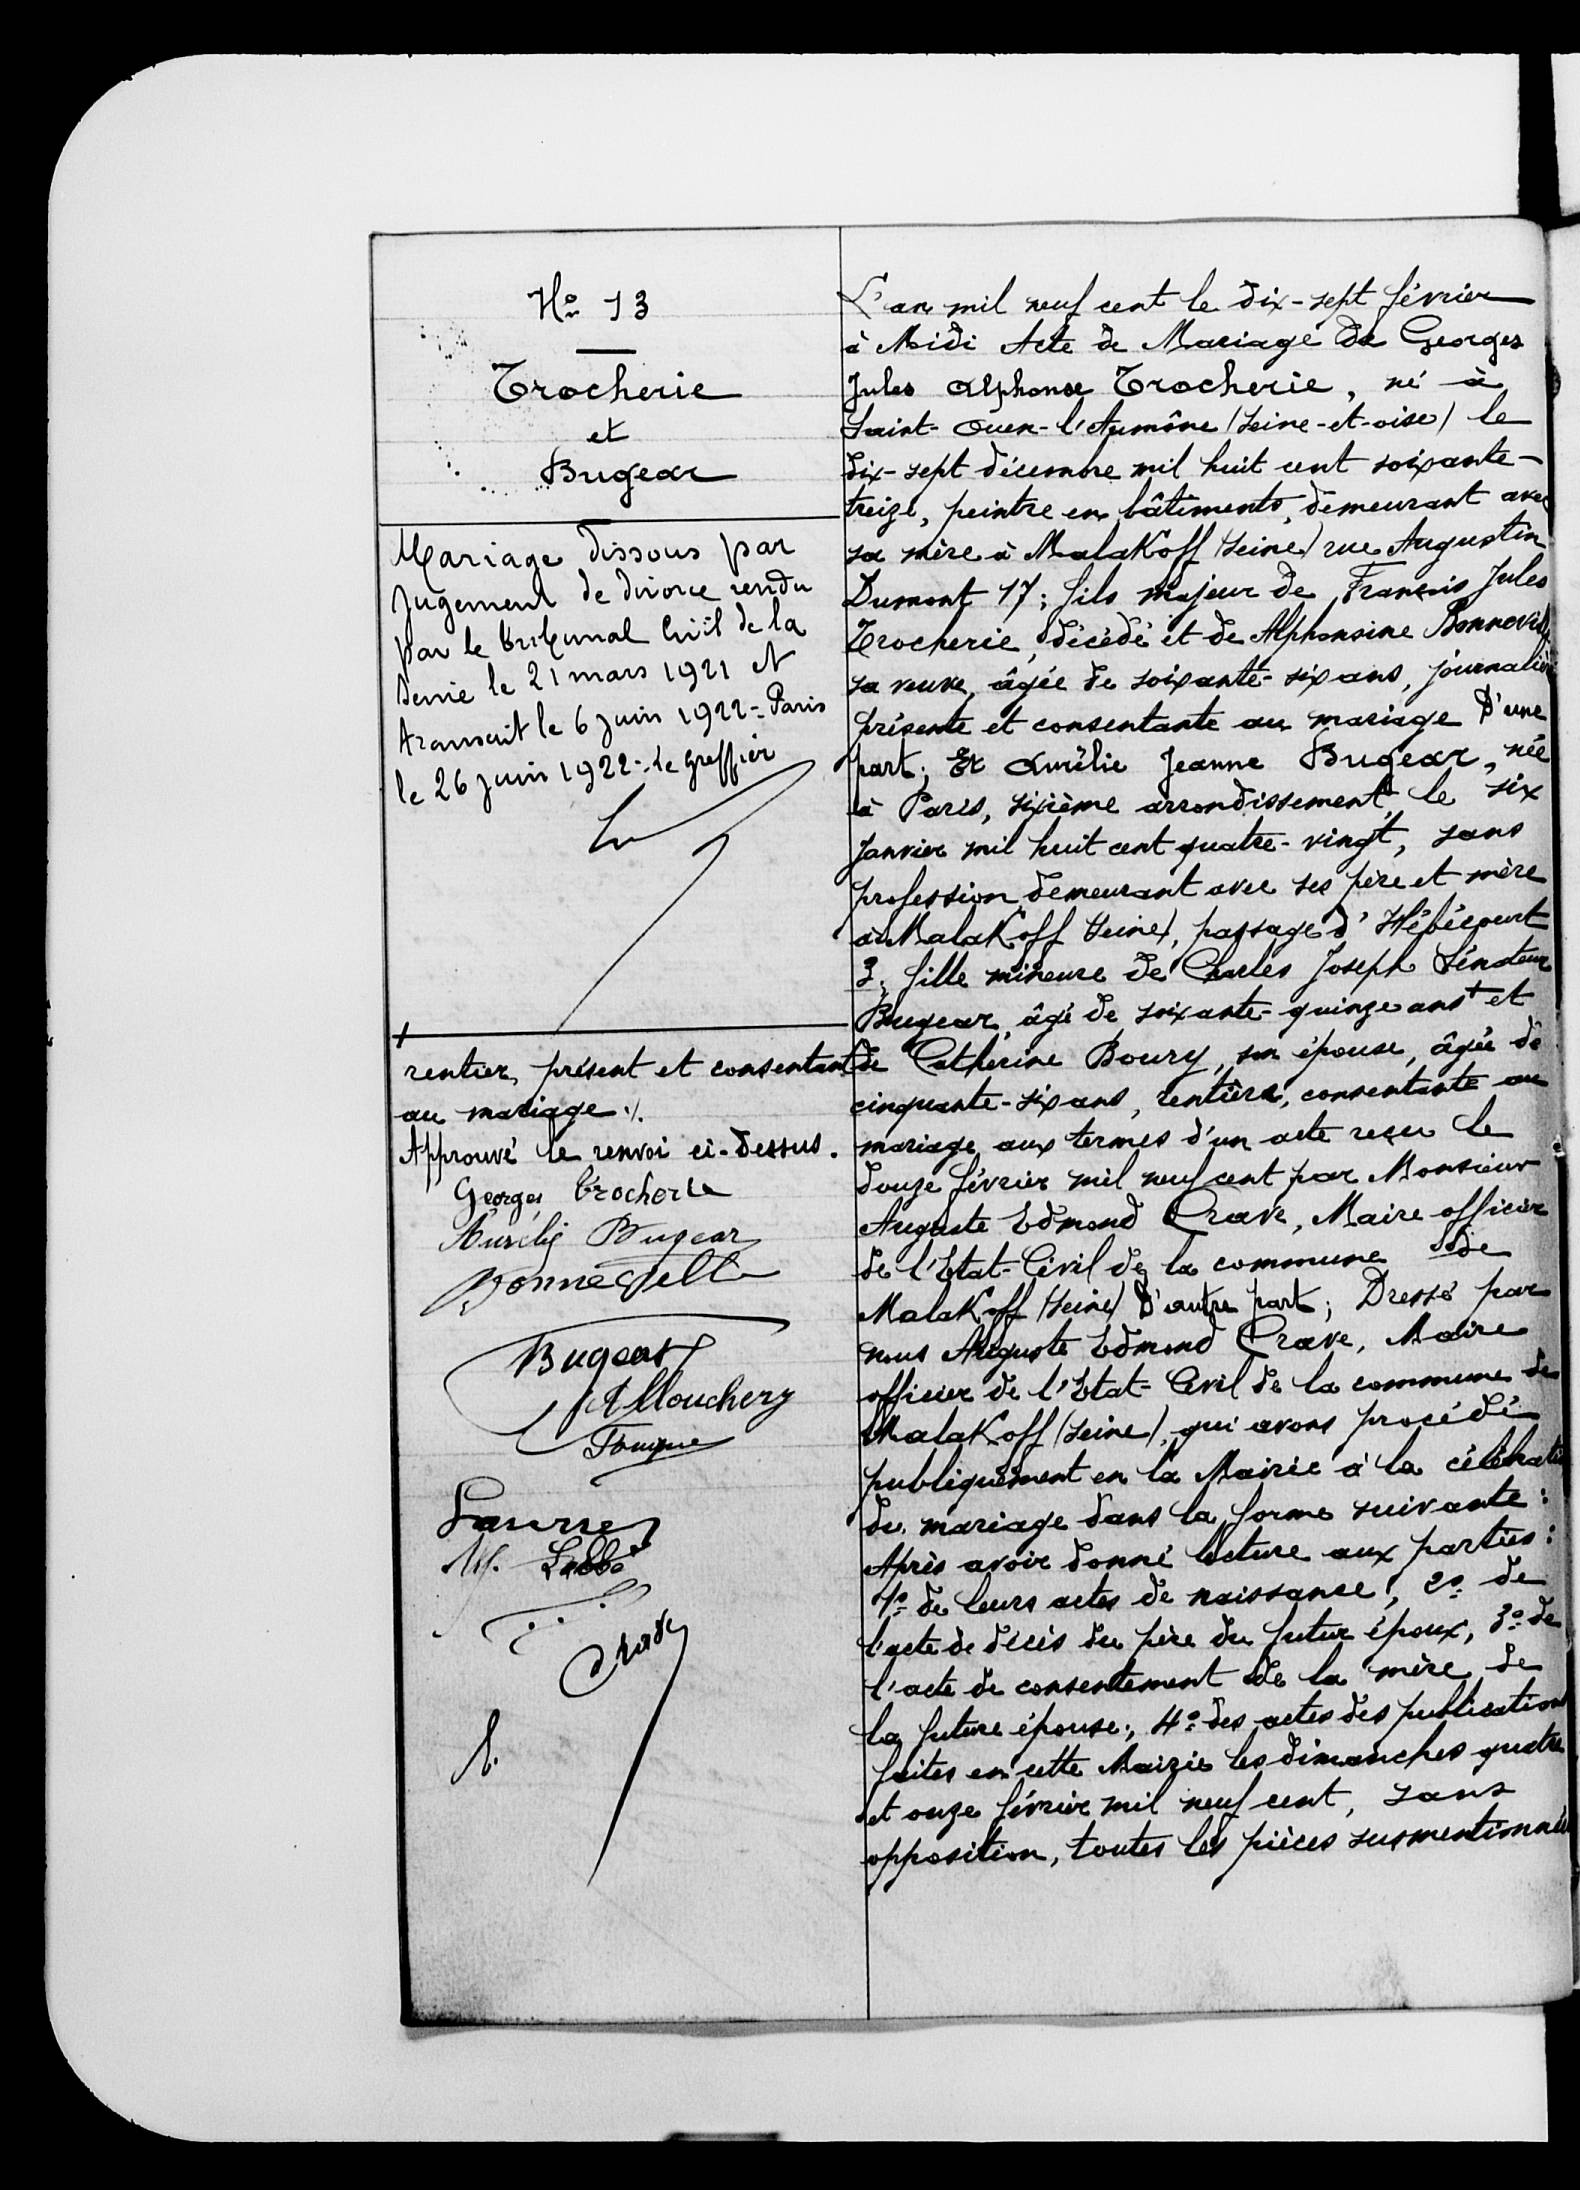N°13
Trocherie
et
Bugear
L'an mil neuf cent le dix-sept février
à Midi Acte de Mariage de Georges
Jules Alphonse Trocherie, né à
Saint-Ouen-l'Aumône (Seine-et-Oise) le
dix-sept décembre mil huit cent soixante -
treize, peintre en bâtiments demeurant avec
sa mère à Malakoff (Seine) rue Augustin
Dumont 17; fils majeur de François Jules
Trocherie, décédé et de Alphonsine Bonnoville,
sa veuve âgée de soixante-six ans, journalière
présente et consentante au mariage D'une
part; Et Amélie Jeanne Bugear née
à Paris, sizième arrondissement le six
Janvier mil huit cent quatre-vingt, sans
profession, demeurant avec ses père et mère
à Malakoff (Seine), passages d'Hébergement
3, fille mineure de Charles Joseph Lénoteur
Bugear, âgé de soixante-quinze ans et
de Catherine Boury, son épouse, âgée de
cinquante-six ans, dentière, consentante au
mariage aux termes d'un acte reçu le
douze février mil neuf cent par Monsieur
Auguste Edmond Crave, Maire officier
de l'Etat-Civil de la commune de
Malakoff (Seine) D'autre part; Dressé par
nous Auguste Edmond Crave, Maire
officier de l'Etat-Civil de la commune de
Malakoff (Seine), qui avons procédé
publiquement en la Mairie à la célébration
du mariage dans la forme suivante:
Après avoir donné lecture aux parties:
1°: de leurs actes de naissance, 2°: de
l'acte de décès du père du futur époux, 3°: de
l'acte de consentement de la mère de
la future épouse; 4°: des actes des publications
faites en cette Mairie les dimanches quatre
et onze février mil neuf cent, sans
opposition, toutes les pièces susmentionnées
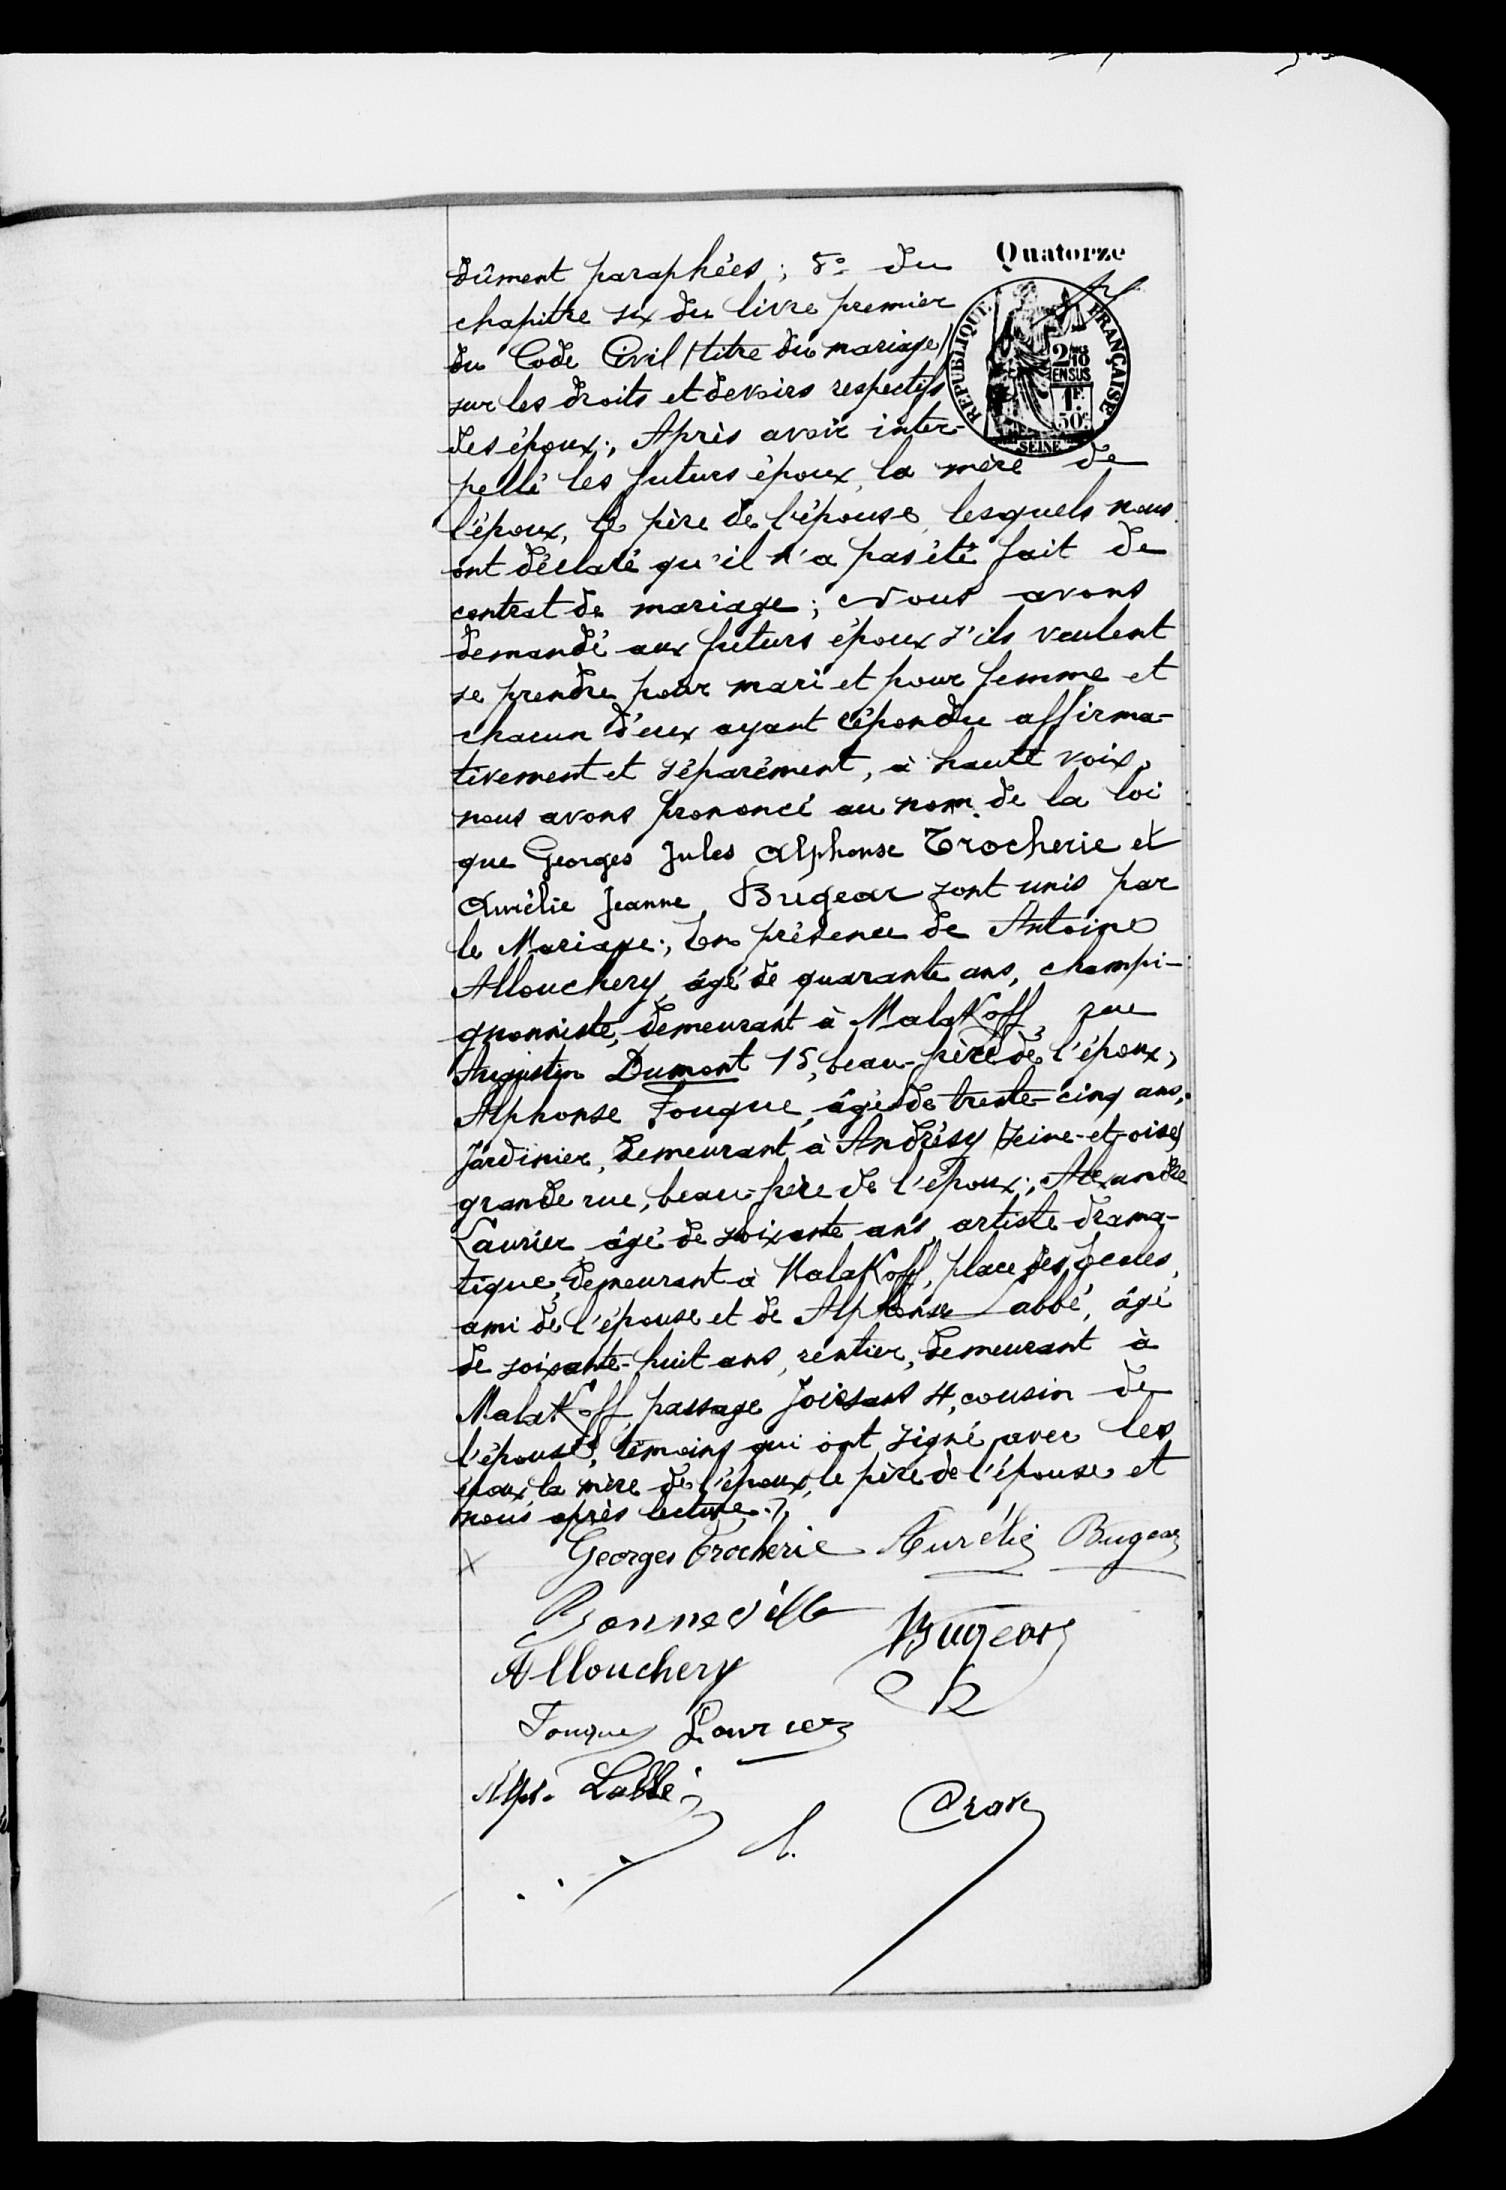dûment paraphées; 5°: du
chapitre six du livre premier
du Code Civil titre du mariage
sur les droits et devoirs respectifs
des époux; Après avoir inter-
pellé les futurs époux, la mère de
l'époux, le père de l'épouse, lesquels nous
ont déclaré qu'il n'a pas été fait de
context de mariage; et nous avons
demandé aux futurs époux s'ils veulent
se prendre pour mari et pour femme et
chacun d'eux ayant répondu affirma-
tivement et séparément, à haute voix.
nous avons prononcé au nom de la loi
que Georges Jules Alphonse Trocherie et
Amélie Jeanne Bugear sont unis par
le Mariage; En présence de Antoine
Allouchery âgé de quarante ans, champi-
gnonniste, demeurant à Malakoff, rue
Augustin Dumont 15, beau-frère de l'époux,
Alphonse Fouque, âgé de trente-cinq ans;
Jardinier, demeurant à Andrésy (Seine-et-oise)
grande rue, beau-frère de l'époux; Alexandre
Laurier âgé de soixante ans artiste drama
tique demeurant à Malakoff, place des Scales
ami de l'épouse et de Alphonse abbé, agé
de soixante-huit ans, rentier, demeurant à
Malakoff, passage Joussant Housin de
l'épouse, témoins qui ont signé avec les
époux, la mère de l'époux, le père de l'épouse et
nous après lecture ./.